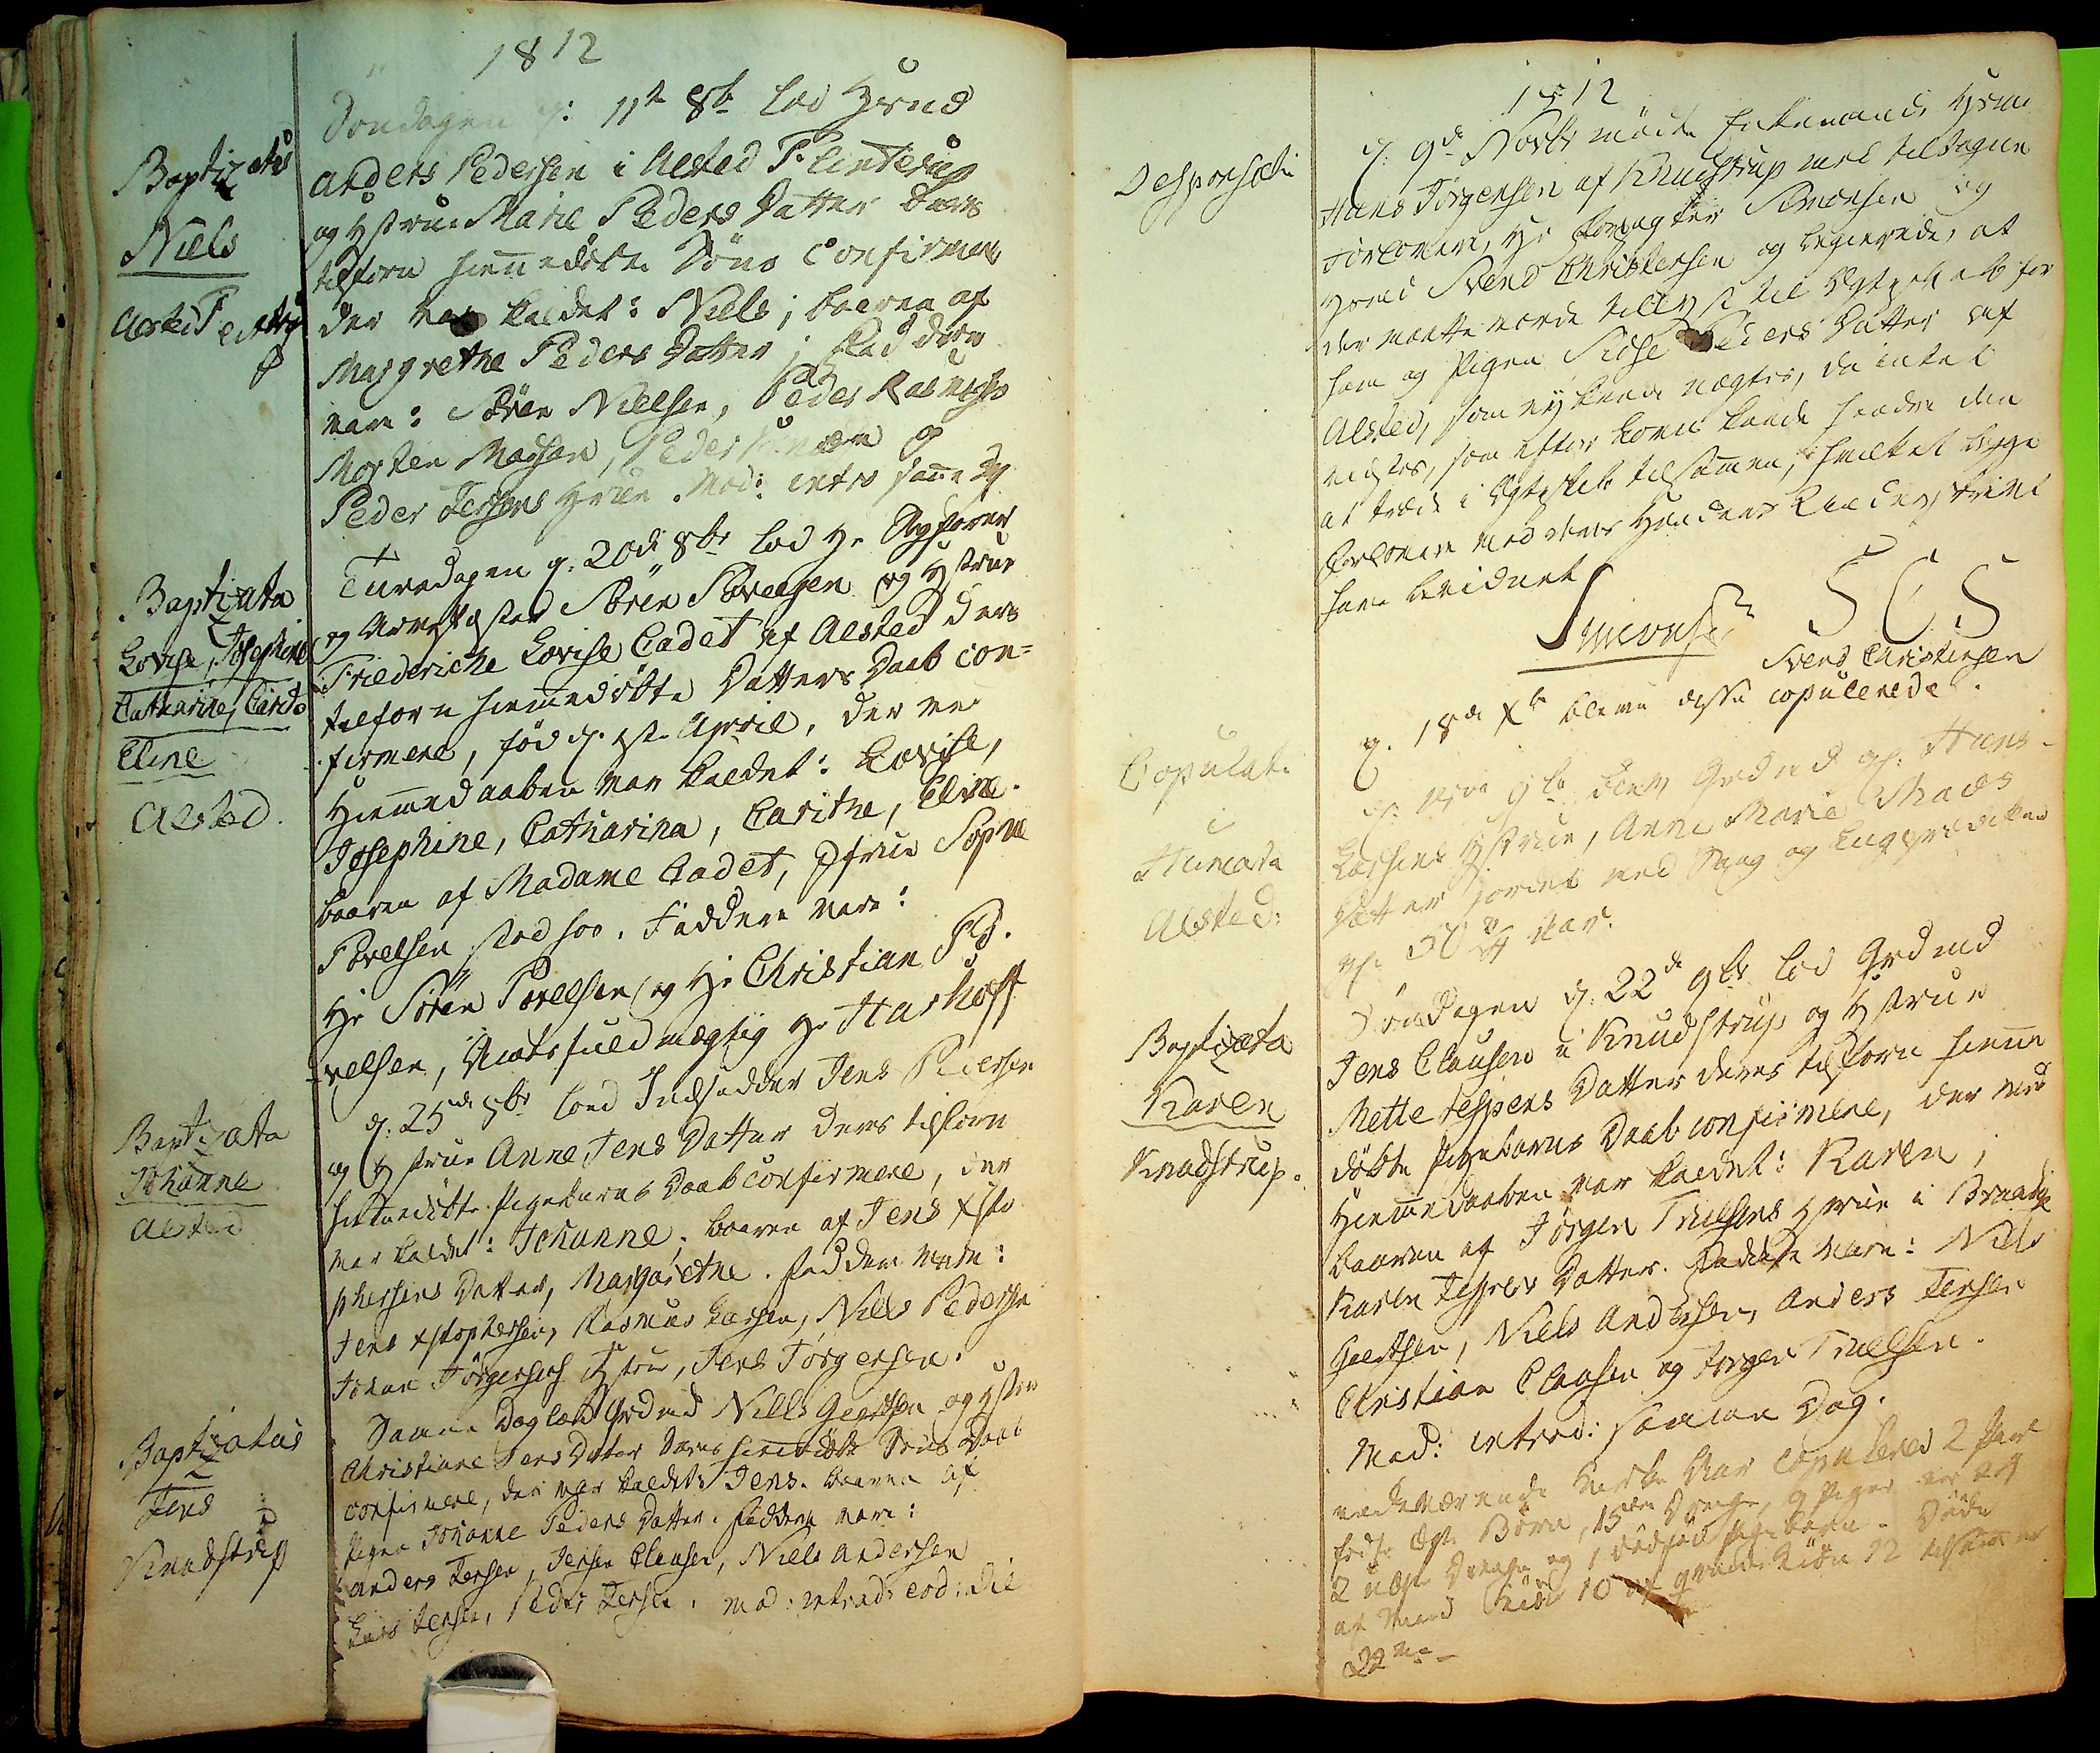Baptizatus
Niels
Alsted Flinterup
Baptizata
Lovise Josephine
Catharine Caritze
Eline
Alsted.
Baptizata
Johanne
Alsted
Baptizatus
Jens
Knudstrup
1812
Sandag d: 11te 8br lod Hsmd
Anders Pedersen i Alsted Flinterup
og Hstrue Marie Peders Datter deres
tilforn hiemmdøbte Søns confirmere
der var kaldet: Niels; baaren af
Margrethe Peders Datter; Faddere
vare: Søren Nielsen, Peder Rasmuss
Morten Madsen, Peder ## og
Peder Jensens Hstrue. Mod: eintrod samme Dag
Tiirsdagen d: 20de 8br lod Hr Sagfører
og Arvefæster Søren Sørensen og Hstrue
Frideriche Lovide Cadet af Alsted deres
tilforn hiemmedøbte Datters Daab con¬
firmere, fød d: 1ste April, der ved
Hiemmedaaben var kaldet: Lovise,
Josephine, Catharina Caritze, Eline.
baareen af Madame Cadet, Jfrue Sophie
Povelsen sted hos. Faddere vare:
Hr Søren Povelsen og Hr Christian Po
velsen, Amtsfuldmægtig Hr Harhoff
d 25de 8br loed Indsidder Jens Pedersen
og Htrue Anne Jens Datter deres tilforn
hiemmedøbte Pigebarns Daab confirmere, der
varng kaldet: Johanne; baaren af Jens Xsto
phersens Datter, Margarethe. Faddere vare:
Jens Xstophersen, Rasmus Larsen, Niels Pedersen
Johan Jørgensens Hstrue, Jens Jørgensen.
Samme Dog loed Grdmd Niels Geertsen og Hstrue
Christiane Jens Datter deres hiemmedøbte Søns Daab
confirmere, der var kaldet: Jens. baaren af
Pigen Johanne Peders Datter. Faddere vare:
Anders Jensen, Jens Clausen, Niels Andersen
Lars Jensen, Peder Jensen. Mod. introd: eod: Die
Desponsati
Copulati
Humata
Alsted
Baptizata
Karen
Knudstrup.
1812
d: 9de Novbr mødte Enkemand Hsmd
Hans Jørgensen af Knudstrup med tiltagne
Forlovere, Hr Forpagter Simonsen og
Hsmd Svend  Christensen og begijerede, at
der maatte vorde tillist til Ægteskab for
ham og Pigen Sidse Peders Datter af
Alsted, som ey kuinde nægtes, da intet
vidstes , som efter Loven kunde hindre dem
at træde i Ægteskab tilsammen, hvilket begge 
Forlevere med deres Hænders Underskrivt
have bevodnet
Simonsen 
S C S6
Svend Christensen.
d: 18de Xbr bleve disse copulerede
d: #### 9br blev Grdmd gl: Hans
Larsens Hstrue, Anne Marie Mads
Datter jordet med Sang og Liigprædiken
gl: d. 50 ## Aar.
Søndagen d: 22de 9br lod Grdmd
Jens Clausen i Knudstrup og Hstrue
Mette Jespers Datter deres tilforn hiemme
Madøbte Pigebarns Daab conformere, der ved
Hiemedaaben var kaldet: Karen;
baaren af Jørgen Truelsens Hstrue i Braabye
Karen Jespers Datter. Faddere vare: Niels
geertsen, Niels Andersens, Anders Jensen
Christian Clausen og Jørgen Truelsen
Mod: introd: samme Dag.
indeværende Kirke Aar copuleret 2 Par
fødte Ægte Børn 15## Drenge, 9 Piger ## ##
2 uægte Drenge og 1 dødfød Pigebarn. Døde
af Mand Kiøn 10 af Qvinde Kiøn 12 tilsammen 
22ma.
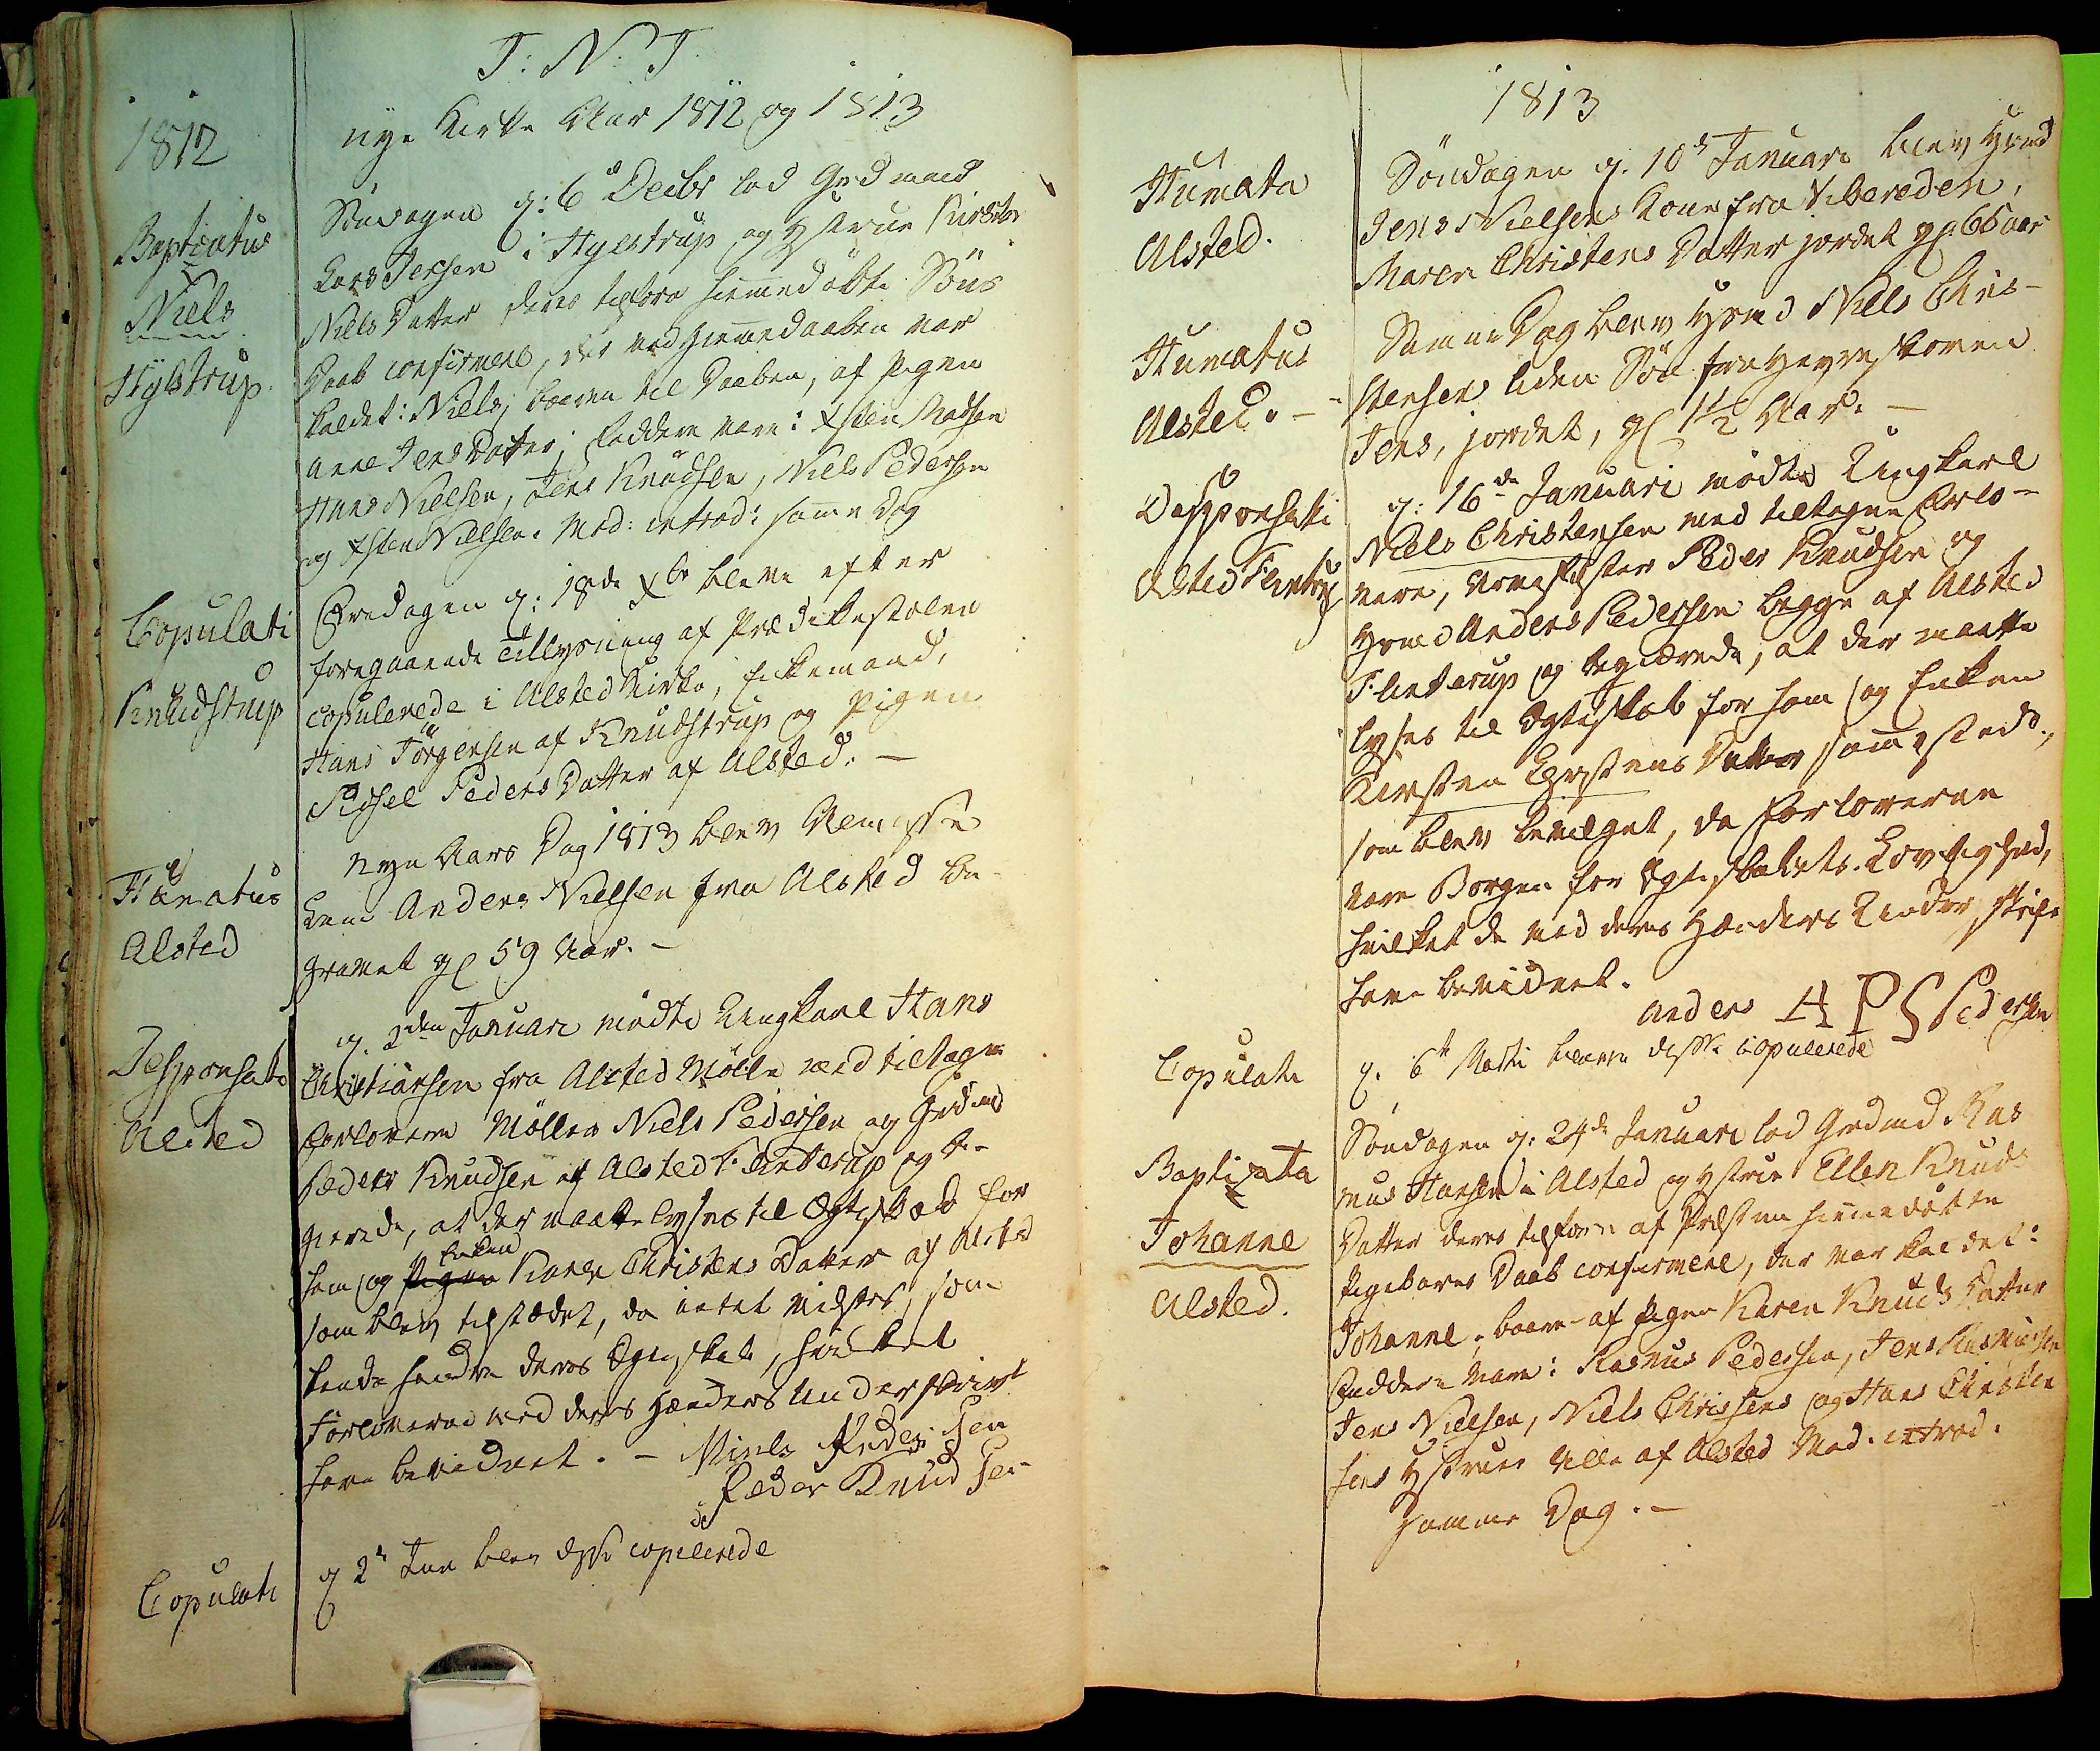1812
Baptizatus
Niels
Hylstrup.
Copulati
Knudstrup
Hanatus
Alsted
Desponsati
Alsted
Copulati
J: N: J.
nye Kirke Aar 1812 og 1813
Søndagen d: 6de Decbr lod Grdmand
Lars Jensen i Hylstrup og Hstrue Kirsten
Niels Datter deres tilforn hiemmedøbte Søns
Daab confirmere, der ved Hiemmedaaben var
kaldet: Niels, baaren til Daaben af Pigen
Anne Jens Datter; Faddere vare: Xsten Madsen
Hans Nielssen, Jens Knudsen, Niels Pedersen
og Xsten Nielsen. Mod: introd: samme Dag
Fredagen d: 18de Xbr bleve efter
foregaaende Tillysning af Prædikestolen
copulerede i Alsted Kirke Enkemand,
Hans Jørgensen af Knudstrup og Pigen
Sidsel Peders Datter af Alsted.
Nye Aars Dag 1813 blev Almisse
lem Anders Nielsen fra Alsted be
gravet gl 59 Aar.
d: 2den Januari mødte Ungkarl Hans
Christiansen fra Alsted Mølle med tiltagne
Forlovere Møller Niels Pedersen og Grdmd
Peder Knudsen af Alsted Flinterup og be¬
gierede, at der maatte lyses til Ægteskab for
Enken
ham og Pigen Karen Christens Datter af Alsted
som blev tilstædet, da antet vidstes, som
kunde hindre deres Ægteskab, hvilket
Forloverne ved deres Hænders Underskrivt
har bevidnet. - Niels Pedersen
Peder Knudsen
d 2## Jan. blev disse copulerede
Humata
Alsted.
Humatus
Alsted.
Desponsati
Alsted Flinterup
Copulati
Baptizata
Johanne
Alsted.
1813
Søndagen d: 10de Januari blev Hsmd
Jens Nielsens Kone fra Viberden
Maren Christens Datter jordet gl: 65 Aar
Samme Dag blev Hsmd Niels Chri¬
stensens liden Søn fra Havreskoven
Jens, jordet, gl 1½ Aar.
d: 16de Januari mødte Ungkarl
Niels Christensen med tiltagne Forlo¬
vere, Arvefæster Peder Knudsen og
Hsmd Anders Pedersen begge af Alsted
Flinterup og begierede, at der maatte
lyses til Ægteskab for ham og Enken
Kirsten Christens Datter sammesteds,
som blev bevilget, da forloverne
vare Borgen for Ægteskabets Lovlighed,
hvilket de ved deres Hænders Underskrift
have bevidnet.
Anders A P S Pedersen
d. 6te Marti bleve disse copulerede
Søndagen d: 24de Januari lod Grdmd Ras
mus Hansen i Alsted og Hstue Ellen Knuds
Datter deres tilforn af Præsten hiemmedøbte
Pigebarns Daab confirmere, der var kaldet:
Johanne; baaren af Pigen Karen Knuds Datter
Faddere vare: Rasmus Pedersen, Jens Rasmussen
Jens Nielssen, Niels Christens og Hans Christen
sens Hstruer Alle af Alsted Mod. introd.
samme Dag